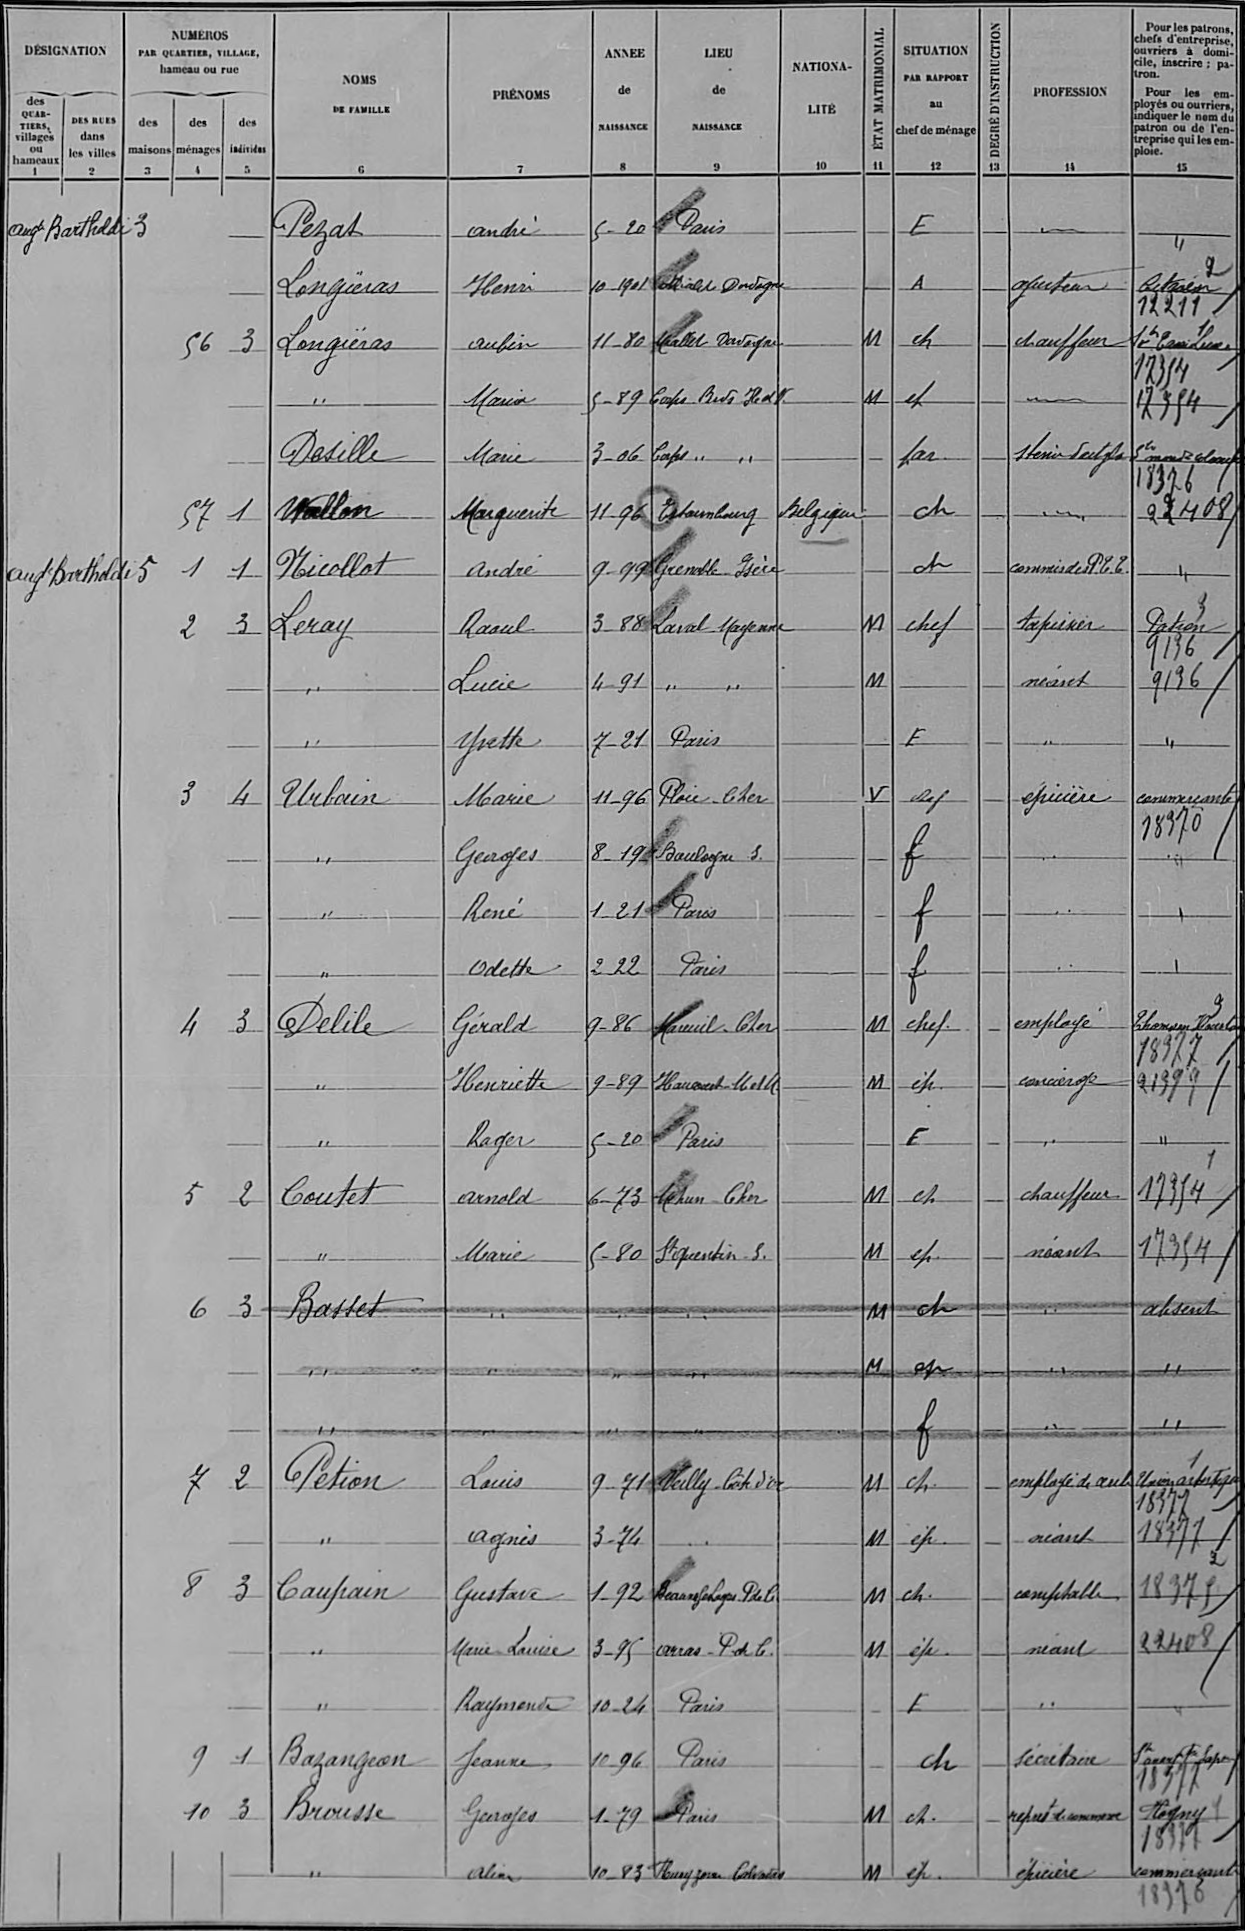Pezat/andré/5 20/Paris/¤/¤/E/¤/¤/¤
Longiéras/Henri/10 1901/Miallet Dordogne/¤/¤/A/¤/ajusteur/Citroen!12211
Longiéras/Aubin/11 80/Miallet Dordogne/¤/M/ch/¤/chauffeur/Ste taxi Luxe!17354
''/Maria/5 89/Corps Nuds Ile et V/¤/M/ch/¤/¤/17354
Desille/Marie/3 06/Corps '' ''/¤/¤/par/¤/sténo dactylo/Ste monde colonne !18326
Wallon/Marguerite/11 96/Estaimbourg/Belgique/¤/ch/¤/¤/22408
Nicollot/André/9 99/Grenoble Isère/¤/¤/ch/¤/commis des PTT/''
Leray/Raoul/3 88/Laval Mayenne/¤/M/chef/¤/tapissier/Patron!9136
''/Lucie/4 91/'' ''/¤/M/¤/¤/néant/9136
''/Yvette/7 21/Paris/¤/¤/E/¤/''/''
Urbain/Marie/11 96/Plou Cher/¤/V/chef/¤/epicière/commercante!18370
''/Georges/8 19/Boulogne s /¤/¤/f/¤/¤/¤
''/René/1 21/Paris/¤/¤/f/¤/¤/¤
''/Odette/2 22/Paris/¤/¤/f/¤/¤/¤
Delile/Gérald/9 86/Mareuil Cher/¤/M/chef /¤/employé/Thomson Lauto!18377
''/Henriette/9 89/Haucourt M et M/¤/M/ép /¤/concierge/21399
''/Roger/5 20/Paris/¤/¤/E/¤/''/''
Coutet/arnold/6 73/Mehun Cher/¤/M/ch/¤/chauffeur/17354
''/Marie/5 80/St Quentin S /¤/M/ep /¤/néant/17354
Basset/¤/¤/¤/¤/M/ch/¤/¤/absent
''/''/''/''/¤/M/ep/¤/''/''
''/''/''/''/¤/¤/f/¤/''/''
Petion/Louis/9 71/Veilly Côte d'or/¤/M/ch /¤/employé de aub/Union artistique!18377
''/Agnès/3 74/¤/¤/M/ép /¤/néant/18377
Caufrain/Gustave/1 92/Beaumetz les Loges P de C /¤/M/ch /¤/comptable/18375
''/Marie-Louise/3 95/arras P de C/¤/M/ép /¤/néant/22408
''/Raymonde/10 24/Paris/¤/¤/F/¤/''/''
Bazangeon/Jeanne/10 96/Paris/¤/¤/ch/¤/secrétaire/St oarf F Lapon!18377
Brousse/Georges/1 79/Paris/¤/M/ch /¤/repret de commerce/Hogny!18379
''/aline/10 83/Fleury orne Calvados/¤/M/ep /¤/épicière/commercant!18976
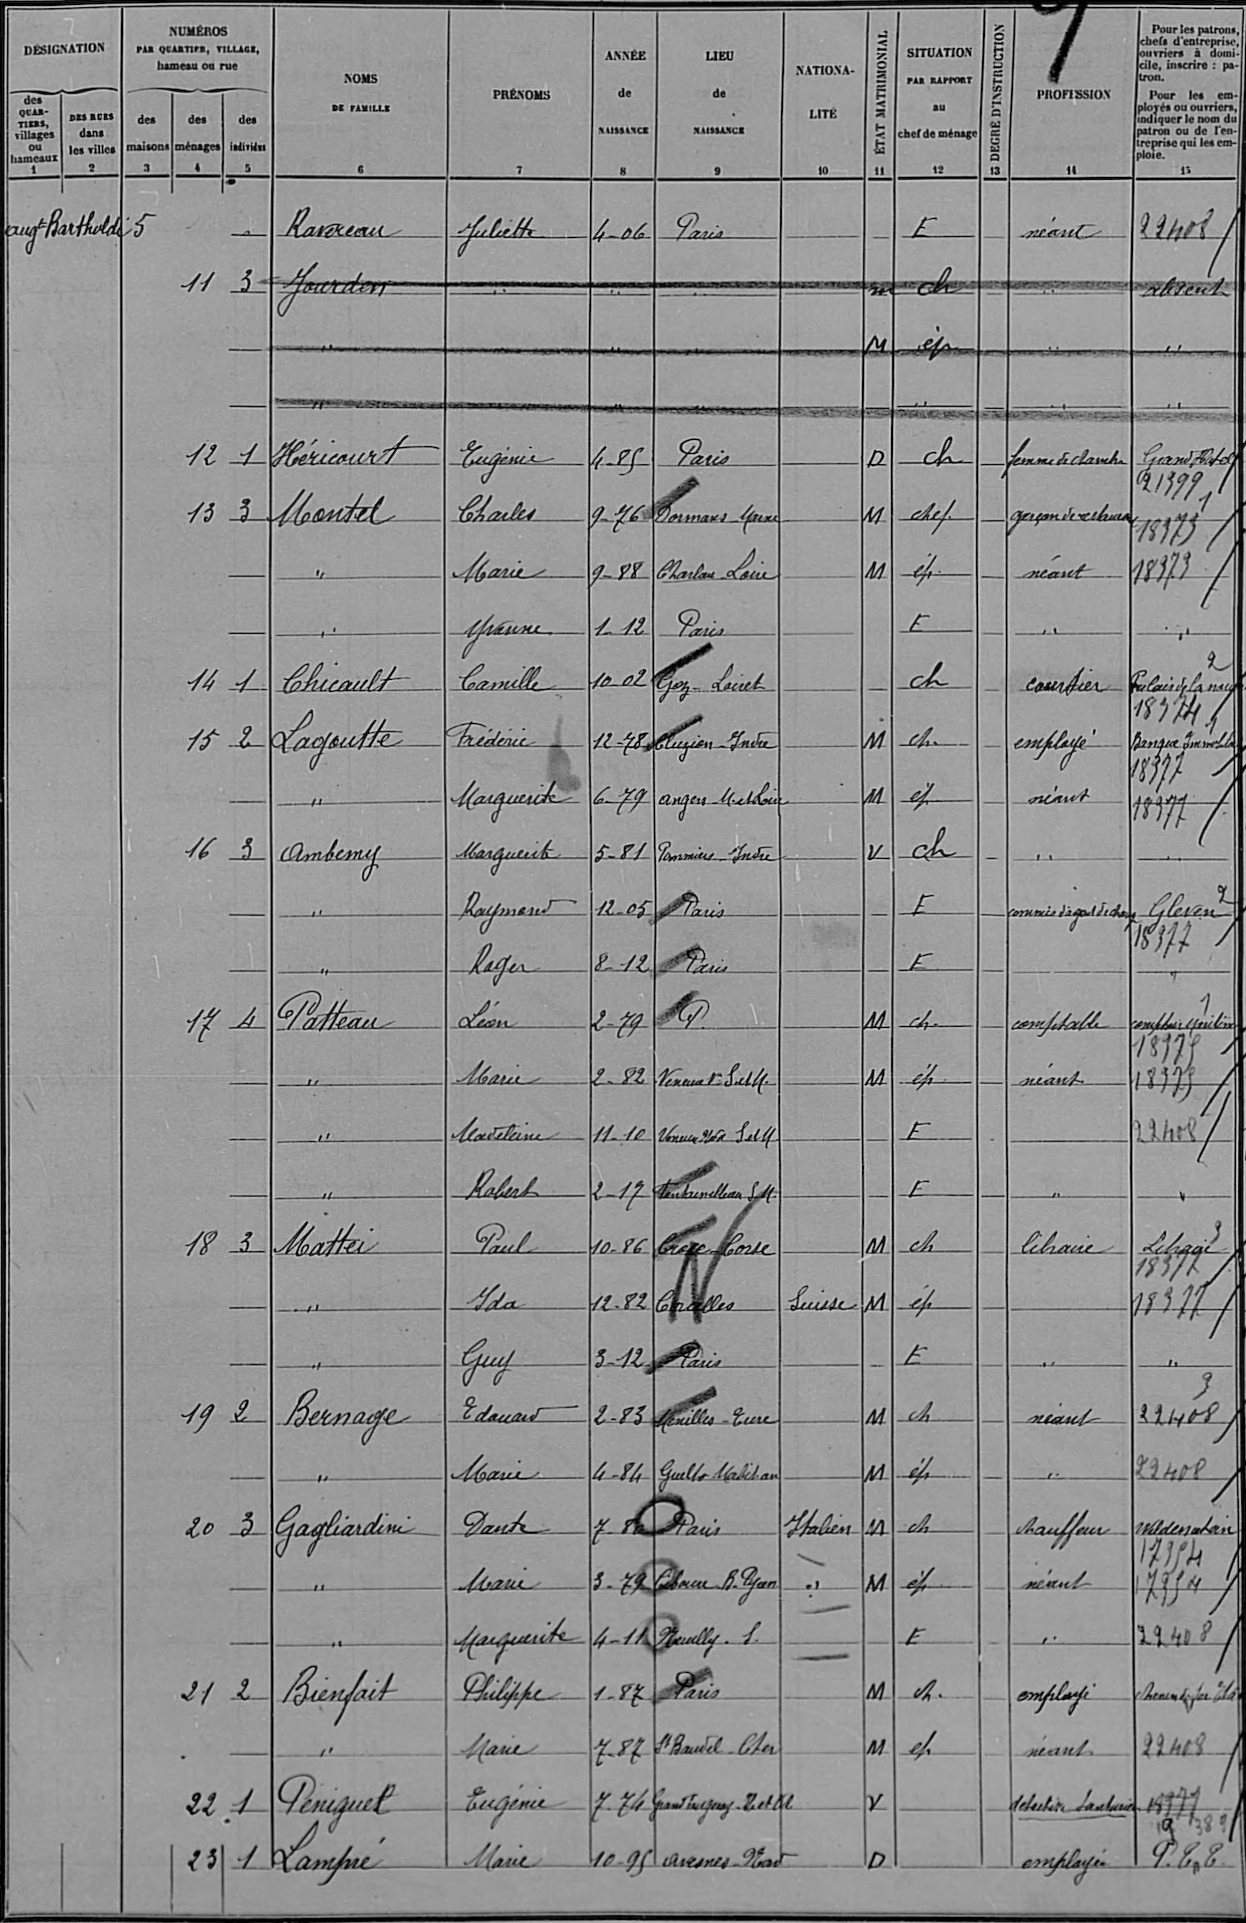Ravereau/Juliette/4 06/Paris/¤/¤/E/¤/néant/22408
Jourden/¤/¤/¤/¤/m/ch/¤/¤/absent
¤/¤/¤/¤/¤/M/ép/¤/¤/¤
¤/¤/¤/¤/¤/¤/¤/¤/¤/¤
Héricourt/Eugénie/4 85/Paris/¤/D/ch/¤/femme de chambre/grand hotel!21399
Montel/Charles/9 76/Dormans Marne/¤/M /chef /¤/garçon de restaurant/18373
¤/Marie/9 88/Charlau Loire/¤/M/ép /¤/néant/18373
¤/Yvonne/1 12/Paris/¤/¤/E/¤/¤/¤
Chicault/Camille/10 02/Goy Loiret/¤/¤/ch/¤/courtier/Palais de la nor!18374
Lagoutte/Frédéric/12 48/Cuzion-Indre/¤/M/ch /¤/employé/banque immobilier!18377
¤/Marguerite/6 79/angers M-et Loire/¤/M/ép/¤/néant/18377
Ambemy/Marguerite/5 81/Pommiers Indre/¤/V/ch/¤/¤/¤
¤/Raymond/12 05/Paris/¤/¤/E/¤/commis d'agent de change/Gleven!18377
¤/Roger/8 12/Paris/¤/¤/E/¤/¤/¤
Patteau/Léon/2 79/P /¤/M/ch /¤/comptable/comptoir unili!18979
¤/Marie/2 82/Veneux S et M/¤/M/ép /¤/néant/18979
¤/Madeleine/11 10/Veneux MdM S et M/¤/¤/E/¤/¤/22408
¤/Robert/2 17/Fontainebleau S M /¤/¤/E/¤/¤/¤
Mattei/Paul/10 86/Croce Corse/¤/M/ch/¤/libraire/Lehacie!18377
¤/Ida/12 82/Crolles/Suisse/M/ép/¤/¤/18377
¤/Guy/3 12/Paris/¤/¤/E/¤/¤/3
Bernage/Edouard/2 83/Menilles Eure/¤/M/ch/¤/néant/22408
¤/Marie/4 84/Guelto Morbihan/¤/M/ép/¤/¤/22408
Gagliardini/Dante/7 80/Paris/Italien/M/ch/¤/chauffeur/Wildenstein!17354
¤/Marie/3 79/Cibourne B Pyren/¤/M/ep/¤/néant/17354
¤/Marguerite/4 11/Neuilly S/¤/¤/E/¤/¤/22408
Bienfait/Philippe/1 87/Paris/¤/M/ch /¤/employé/chemin de fer Etat
¤/Marie/7 87/St Baudel Cher/¤/M/ep/¤/néant/22408
Peniguel/Eugénie/7 74/Grand Turgeuy Ile Et Cl/¤/V/¤/¤/détective tanturier/18377!19389
Lampré/Marie/10 95/avesnes Nord/¤/D/¤/¤/employée/P T T